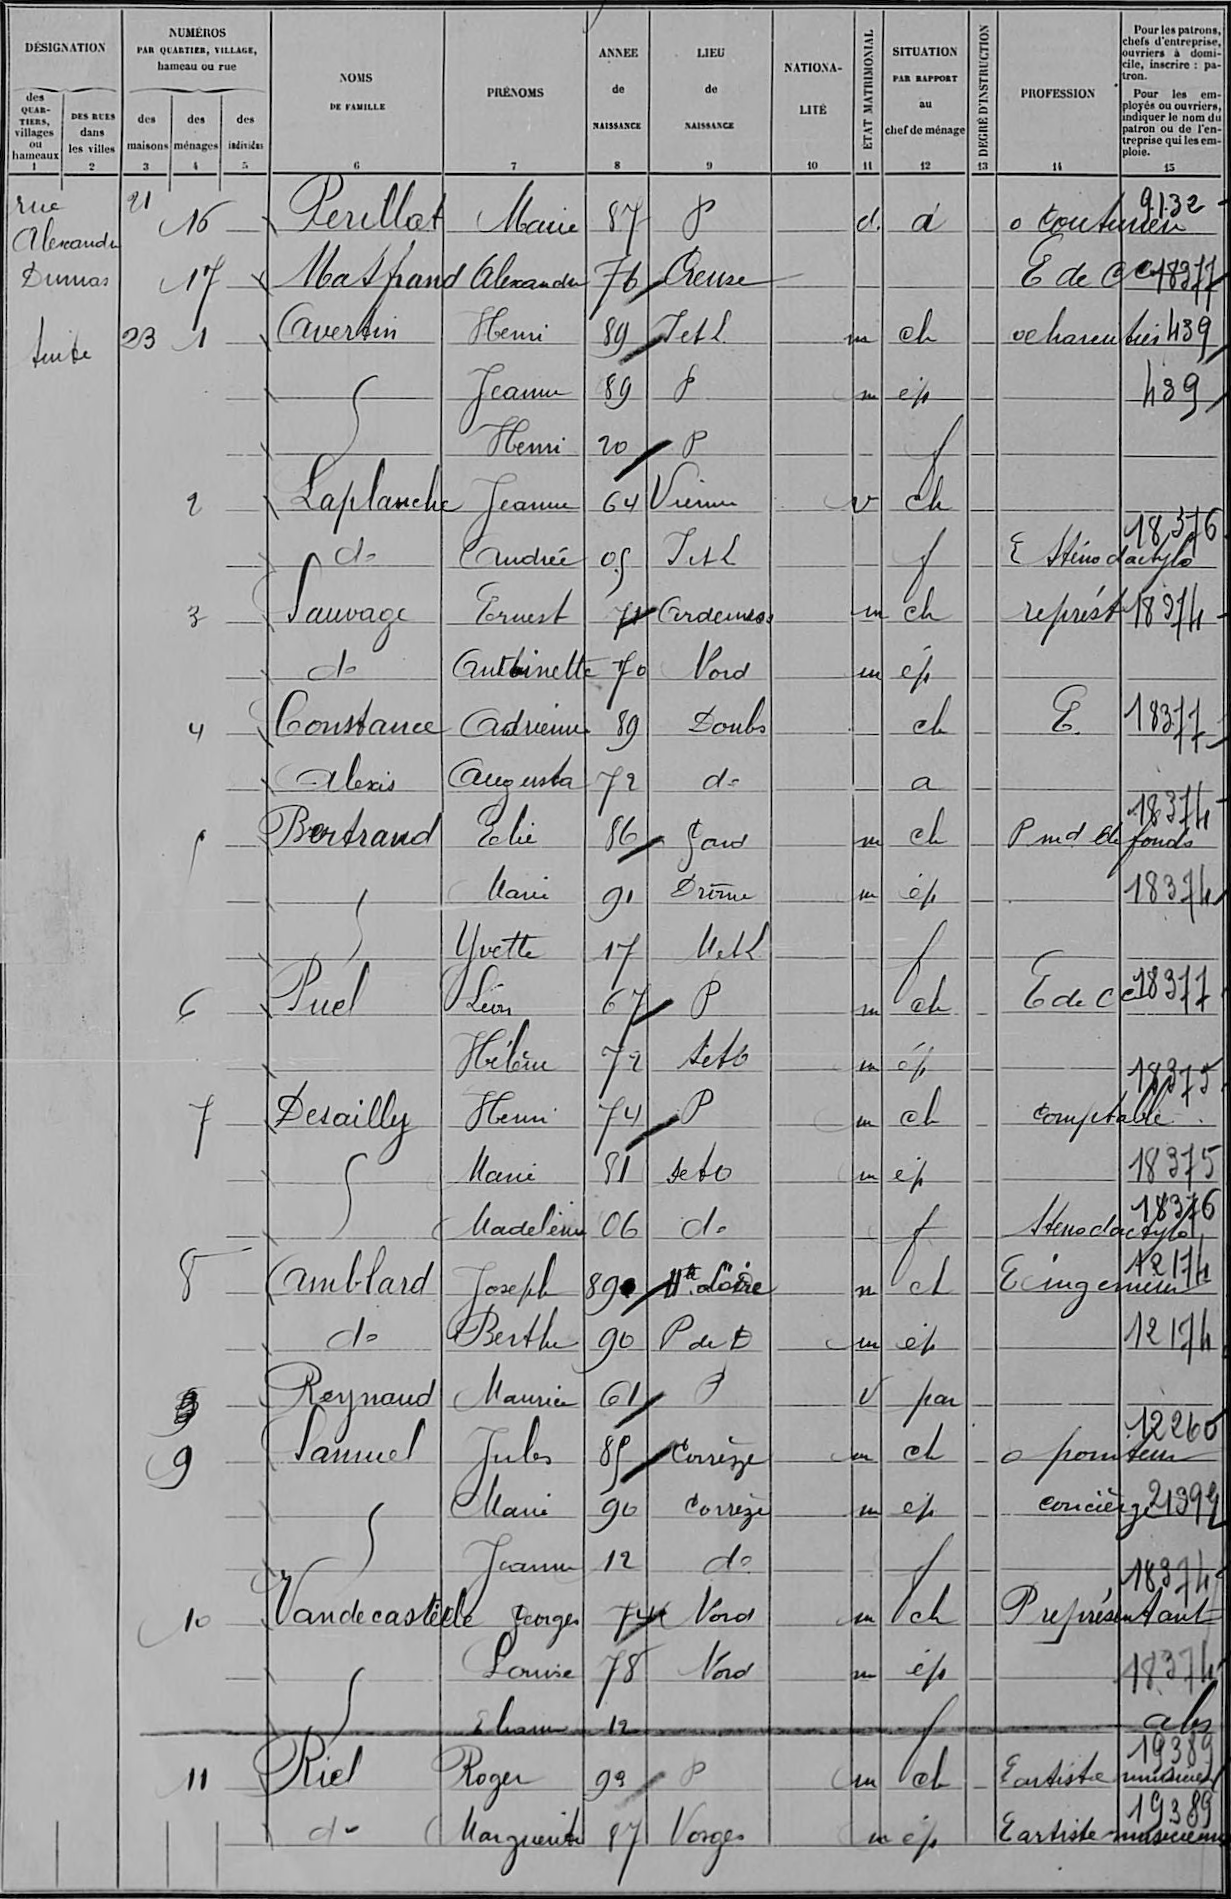Perillat/Marie/87/P/¤/d /d/¤/o couturier/?9132
Masfrand/alexandre/76/Creuse/¤/¤/¤/¤/E de Cce/18377
Averlin/Henri/89/I et L /¤/m/ch/¤/o charentier/439
¤/Jeanne/89/P/¤/m/ép/¤/¤/439
¤/Henri/20/P/¤/¤/f/¤/¤/¤
Laplanche/Jeanne/64/Vienne/¤/V/ch/¤/¤/¤
d°/Andrée/05/I et L/¤/¤/f/¤/E sténo dactylo/?18376
Sauvage/Ernest/71/Ardennes/¤/m/ch/¤/représt/18274
d°/Antoinette/70/Nord/¤/m/ép/¤/¤/¤
Constance/Adrienne/89/Doubs/¤/¤/ch/¤/E /18377
Alexis/Augusta/72/d°/¤/¤/a/¤/¤/¤
Bertrand/Elie/86/Gard/¤/m/ch/¤/P md de fonds/?18374
¤/Marie/91/Drôme/¤/m/ép/¤/¤/18374
¤/Yvette/17/M et L/¤/¤/f/¤/¤/¤
Puel/Léon/67/P/¤/m/ch/¤/E de Ce/18377
¤/Hélène/72/S et O/¤/m/ép/¤/¤/18375
Desailly/Henri/74/P/¤/m/ch/¤/comptable/¤
¤/Marie/81/S et O/¤/m/ép/¤/¤/18375
¤/Madeleine/06/d°/¤/¤/f/¤/steno dactylo/18376
Amblard/Joseph/89/Hte Loire/¤/m/ch/¤/E ingenieur/?12174
d°/Berthe/90/P de D/¤/m/ép/¤/¤/12174
Reynaud/Maurice/61/P/¤/V/par/¤/¤/¤
Samuel/Jules/85/Corrèze/¤/m/ch/¤/o pointeur/?12266
¤/Marie/90/Corrèze/¤/m/ép/¤/concierge/21399
¤/Jeanne/12/d°/¤/¤/f/¤/¤/18374
Vandecastede/Georges/74/Nord/¤/m/ch/¤/P représentant/¤
¤/Perrine/78/Nord/¤/m/ép/¤/¤/18374
¤/Elianne/12/¤/¤/¤/f/¤/¤/abs
Riel/Roger/99/P/¤/m/ch/¤/e artiste musicien/?19389
d°/Marguerite/87/Vosges/¤/m/ch/¤/E artiste musicienne/?18389
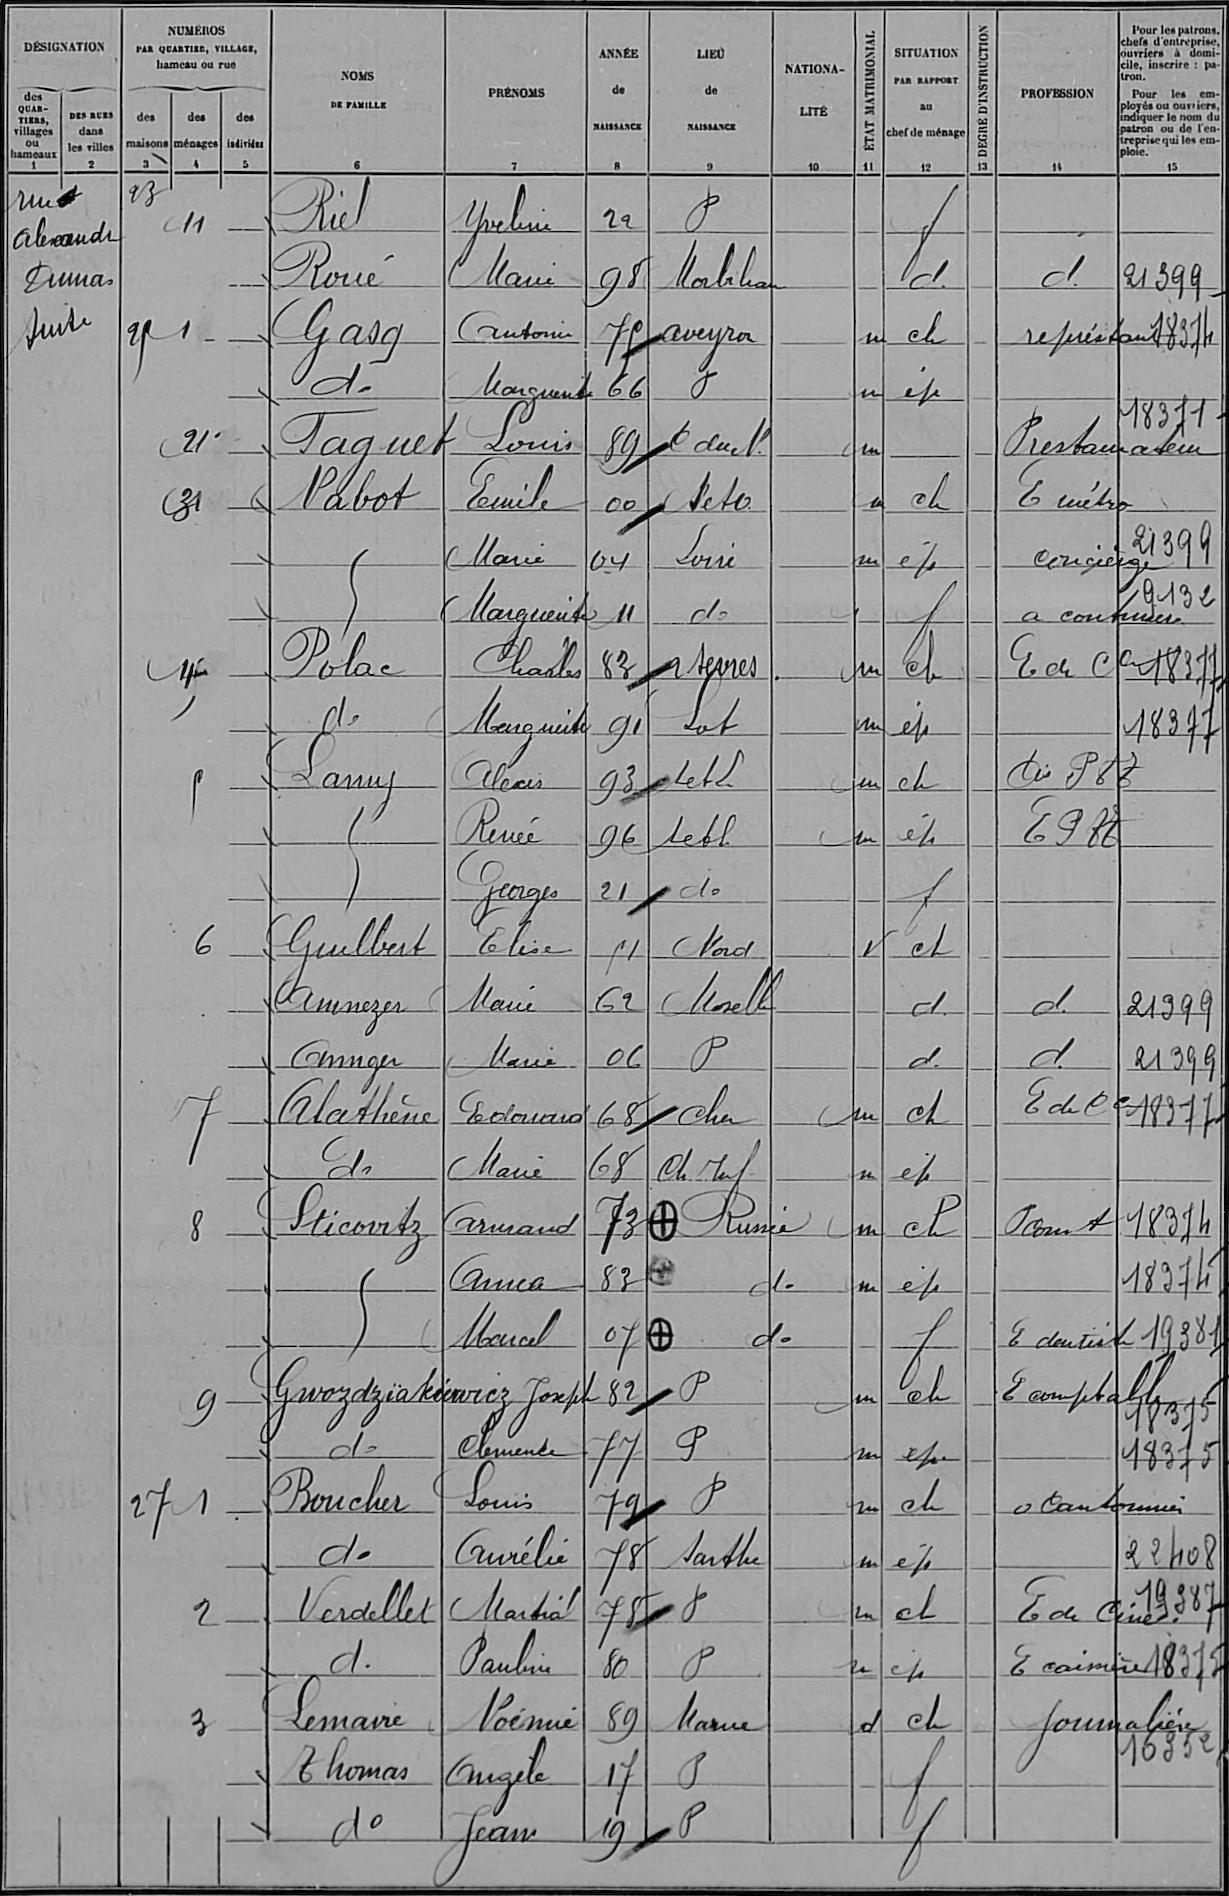Riel/Yveline/22/P/¤/¤/f/¤/¤/¤
Roué/Marie/98/Morbihan/¤/¤/d /¤/d /21399
Gasg/Antoine/75/aveyron/¤/m/ch/¤/représentant/18374
d°/Marguerite/66/P/¤/m/ép/¤/¤/¤
Paquet/Louis/89/C du N /¤/m/¤/¤/Restaurateur/?18371
Nabot/Emile/00/S et O/¤/m/ch/¤/E métro/¤
¤/Marie/04/Loire/¤/m/ép/¤/concierge/?21399
¤/Marguerite/11/d°/¤/¤/f/¤/a couturiere/?9132
Polac/Charles/83/2 Sevres/¤/m/ch/¤/E du Cce/18377
d°/Marguerite/91/Lot/¤/m/ép/¤/¤/18377
Lamy/Alexis/93/L et G/¤/m/ch/¤/Cis PTT/¤
¤/Renée/96/L et G/¤/m/ép/¤/E PTT/¤
¤/Georges/21/d°/¤/¤/f/¤/¤/¤
Guilbert/Elise/51/Nord/¤/V/ch/¤/¤/¤
Amnezer/Marie/62/Moselle/¤/¤/d /¤/d /21399
Amnger/Marie/06/P/¤/¤/d /¤/d /21399
Alathène/Edouard/68/Cher/¤/m/ch/¤/E de Cce/18377
d°/Marie/68/Ch Inf/¤/m/ép/¤/¤/¤
Sticovitz/Armand/73/Russie/¤/m/ch/¤/P comt/18374
¤/Anna/83/d°/¤/m/ép/¤/¤/18374
¤/Marcel/07/d°/¤/¤/f/¤/E dentiste/19381
Gwozdzïakiewicz/Joseph/82/P/¤/m/ch/¤/E comptable/!18375
d°/Clemence/77/P/¤/m/ép /¤/¤/18375
Boucher/Louis/79/P/¤/m/ch/¤/o Cantonnier/¤
d°/Aurélie/78/Sarthe/¤/m/ép/¤/¤/22408
Verdellet/Martial/78/P/¤/m/ch/¤/E de Ciné/?19387
d°/Pauline/80/P/¤/m/ch/¤/E caissière/18375
Lemaire/Noémie/89/Marne/¤/d/ch/¤/journalière/!16352
Thomas/Angèle/17/P/¤/¤/f/¤/¤/¤
d°/Jean/19/P/¤/¤/f/¤/¤/¤